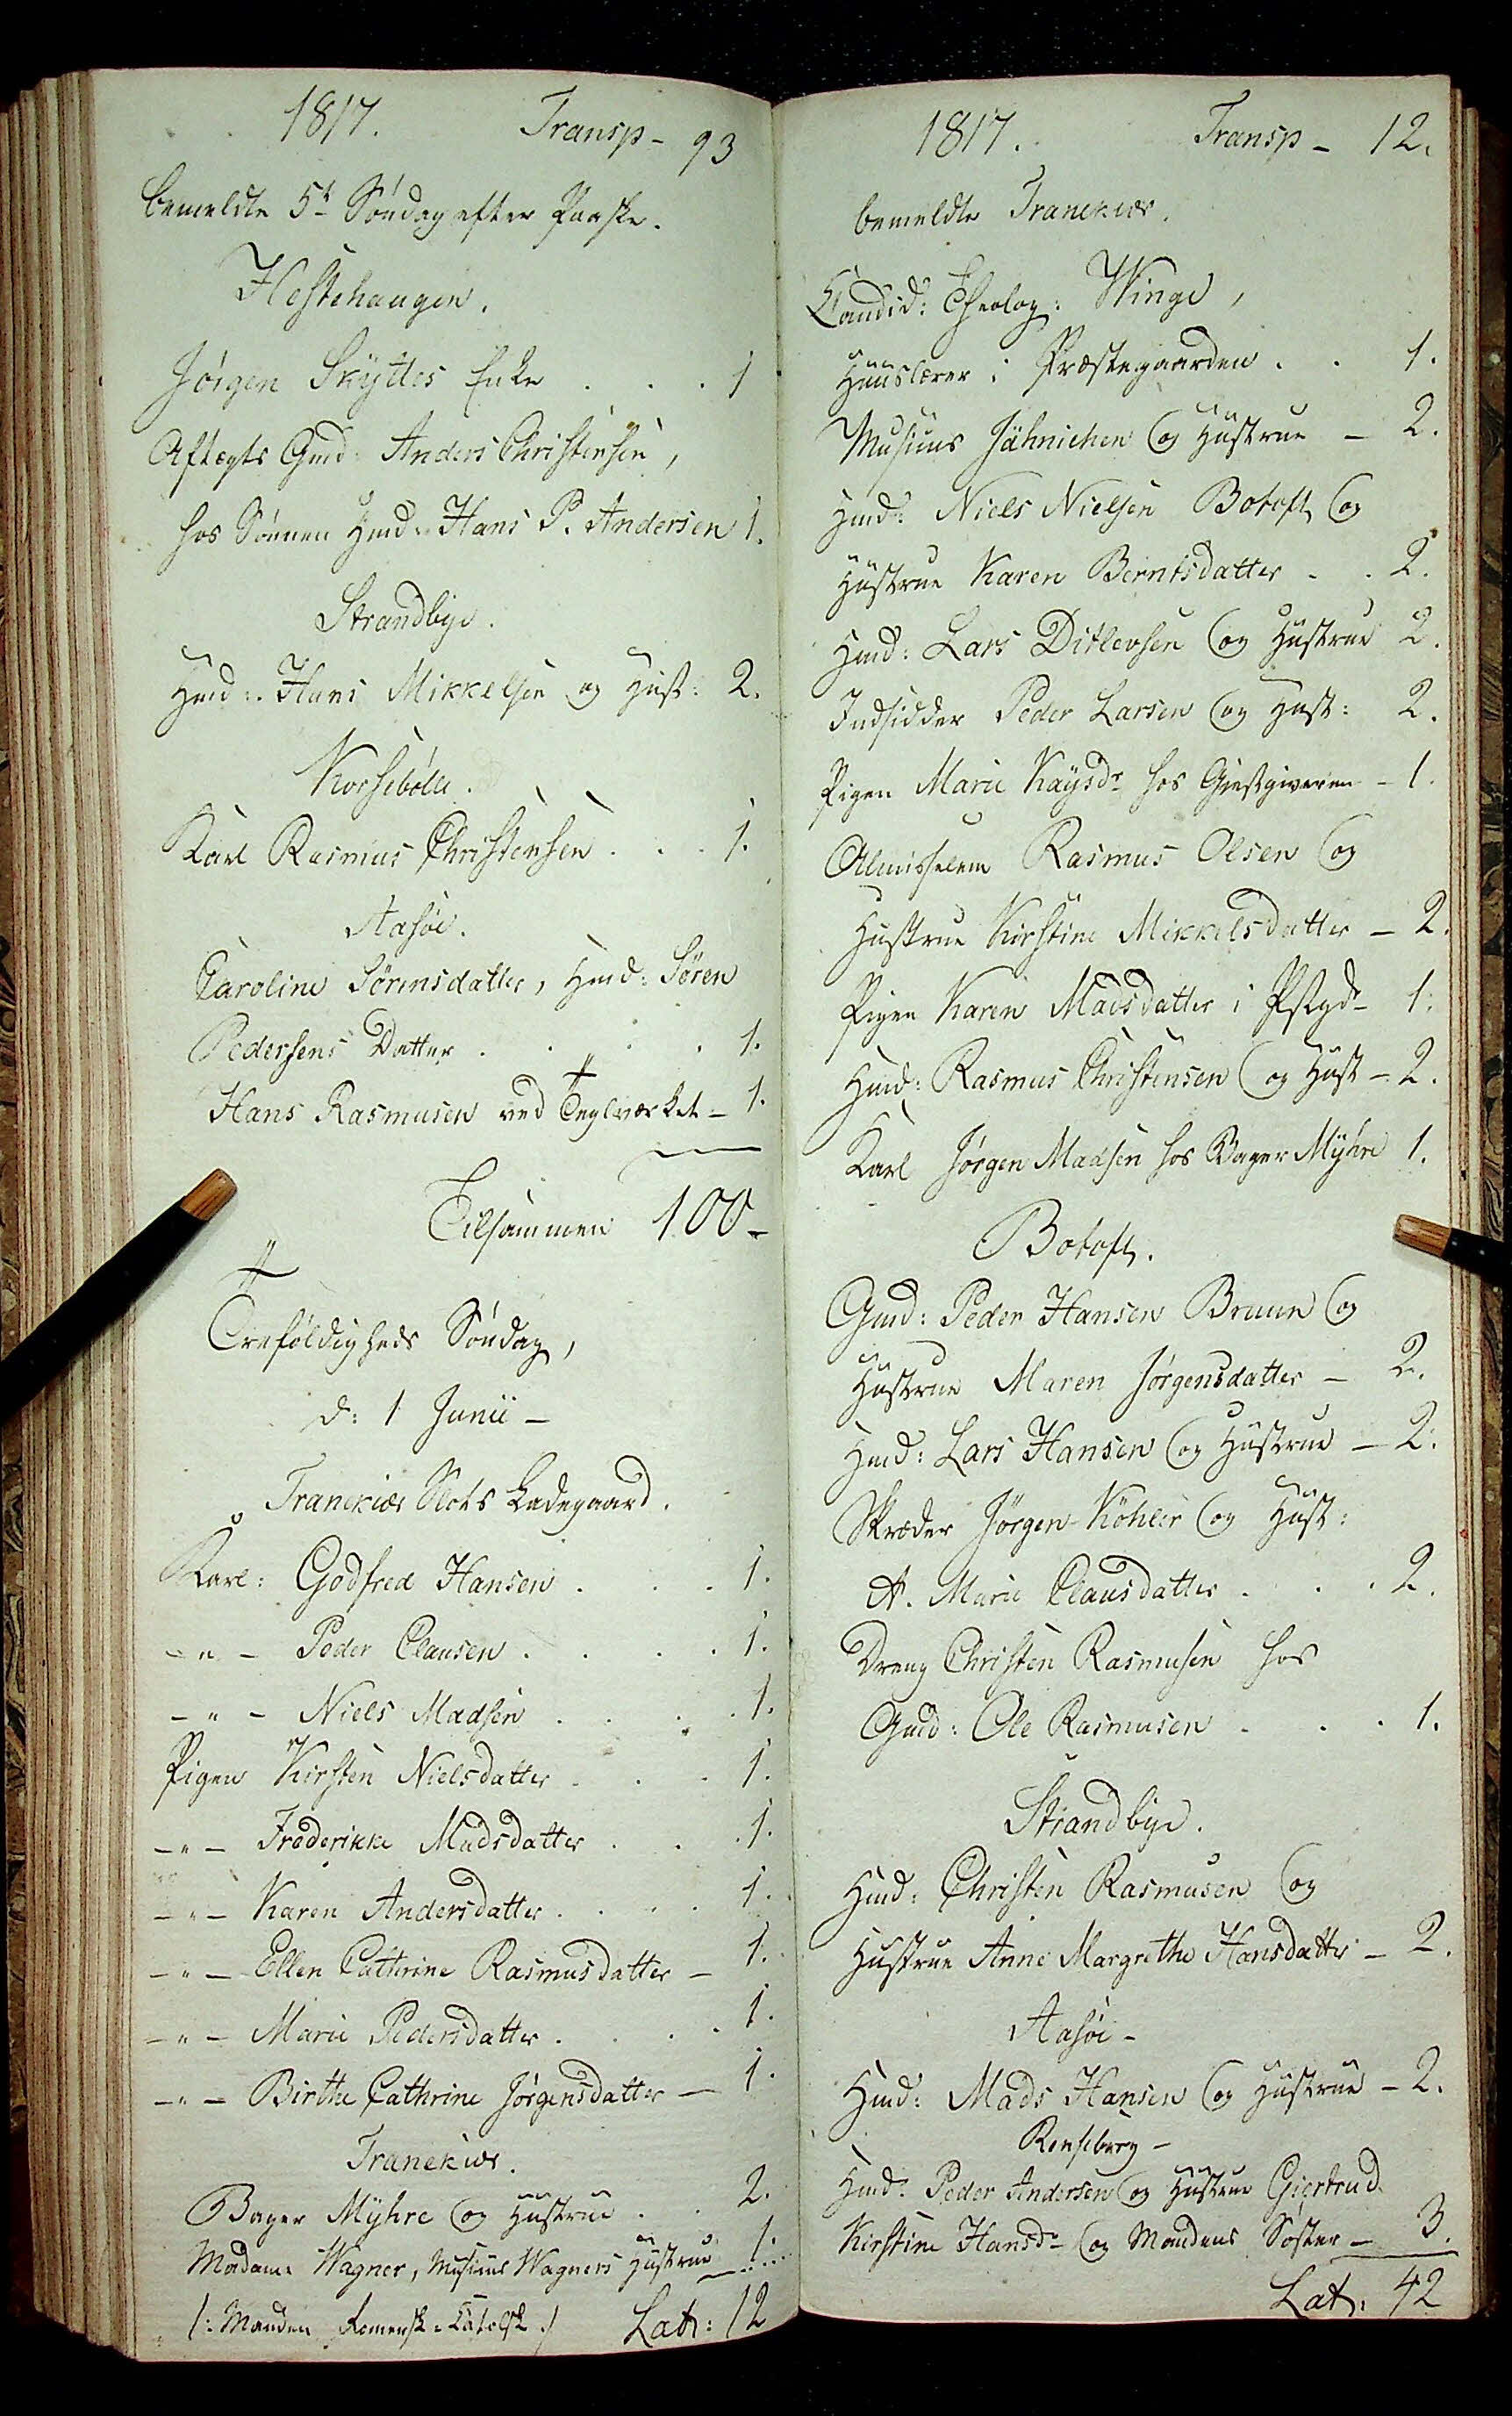1817.
Transp - 93
bemeldte 5te Søndag efter Paaske.
Hestehaugen.
Jørgen Skyttes Enke . . . 1
Aftægts Gmd. Anders Christensen
hos Sønnen Hmd: Hans P. Andersen 1.
Strandnye.
Hmd: Hans Mikkelsen og Hust: 2.
Korsebølle.
Karl Rasmus Christensen . . .1.
Aasøe.
Caroline Sørnsdatter, Hmd: Søren
Pedersens Datter . . . .1.
Hans Rasmusen ved Teglværket - 1.
Tilsammen 100-
Trefoldigheds Søndag
d: 1 Junii-
Tranekiær Slots Ladegaard.
Karl Godfred Hansen . . . 1.
-"- Peder Clausen . . . . 1.
-"- Niels Madsen . . . . 1.
Pigen Kirsten Nielsdatter . . . 1.
-"- Friderikke Madsdatter . . .1.
-"- Karen Andersdatter . . . . 1.
-"- Ellen Cathrine Rasmusdatter - 1.
-"- Marie Pedersdatter . . . . 1.
-"- Birthe Cathrine Jørgensdatter - 1.
Tranekiær
Bager Myhre og Hustrue . . 2.
Madame Wagner, Musicus Wagers Hustrue 1.
/: Manden Romersk Catolsk ./ Lat: 12
1817.
Transp - 12.
bemeldte Tranekiær
Candid: Theolog: Winge
Huuslærer i Præstegaarden . . 1.
Musicus Jahnichen og Hustrue - 2.
Hmd: Niels Nielsen Botoft og
Hustrue Karen Berntsdatter . . 2.
Hmd: Lars Ditlevsen og Hustrue 2.
Indsidder Peder Larsen og Hust: 2.
Pigen Marie Kaysdr hos Giestgiveren - 1.
Almisselem Rasmus Olsen og
Hustrue Kirstine Mikkelsdatter - 2
Pigen Karen Madsdatter i Pstgdr 1:
Hmd: Rasmus Christensen og Hust - 2.
Karl Jørgen Madsen hos Bager Muhre 1.
Botoft.
Gmd: Peder Hansens Bruun og
Hustrue Maren Jørgensdatter - 2.
Hmd: Lars Hansen og Hustrue - 2.
Skrædder Jørgen Køhler og Hust:
A. Marie Clausdatter . . . 2.
Dreng Christen Rasmusen hos
Gmd: Ole Rasmusen . . . 1.
Strandbye.
Hmd: Christen Rasmusen og
Hustrue Anne Margrethe Hansdatter - 2
Aasøe.
Hmd: Mads Hansens og Hustrue - 2.
Renseberg
Hmd: Peder Andersen og Hustrue Giertrud.
Kirstine Hansdr og Mandens Søster - 3
Lat: 42
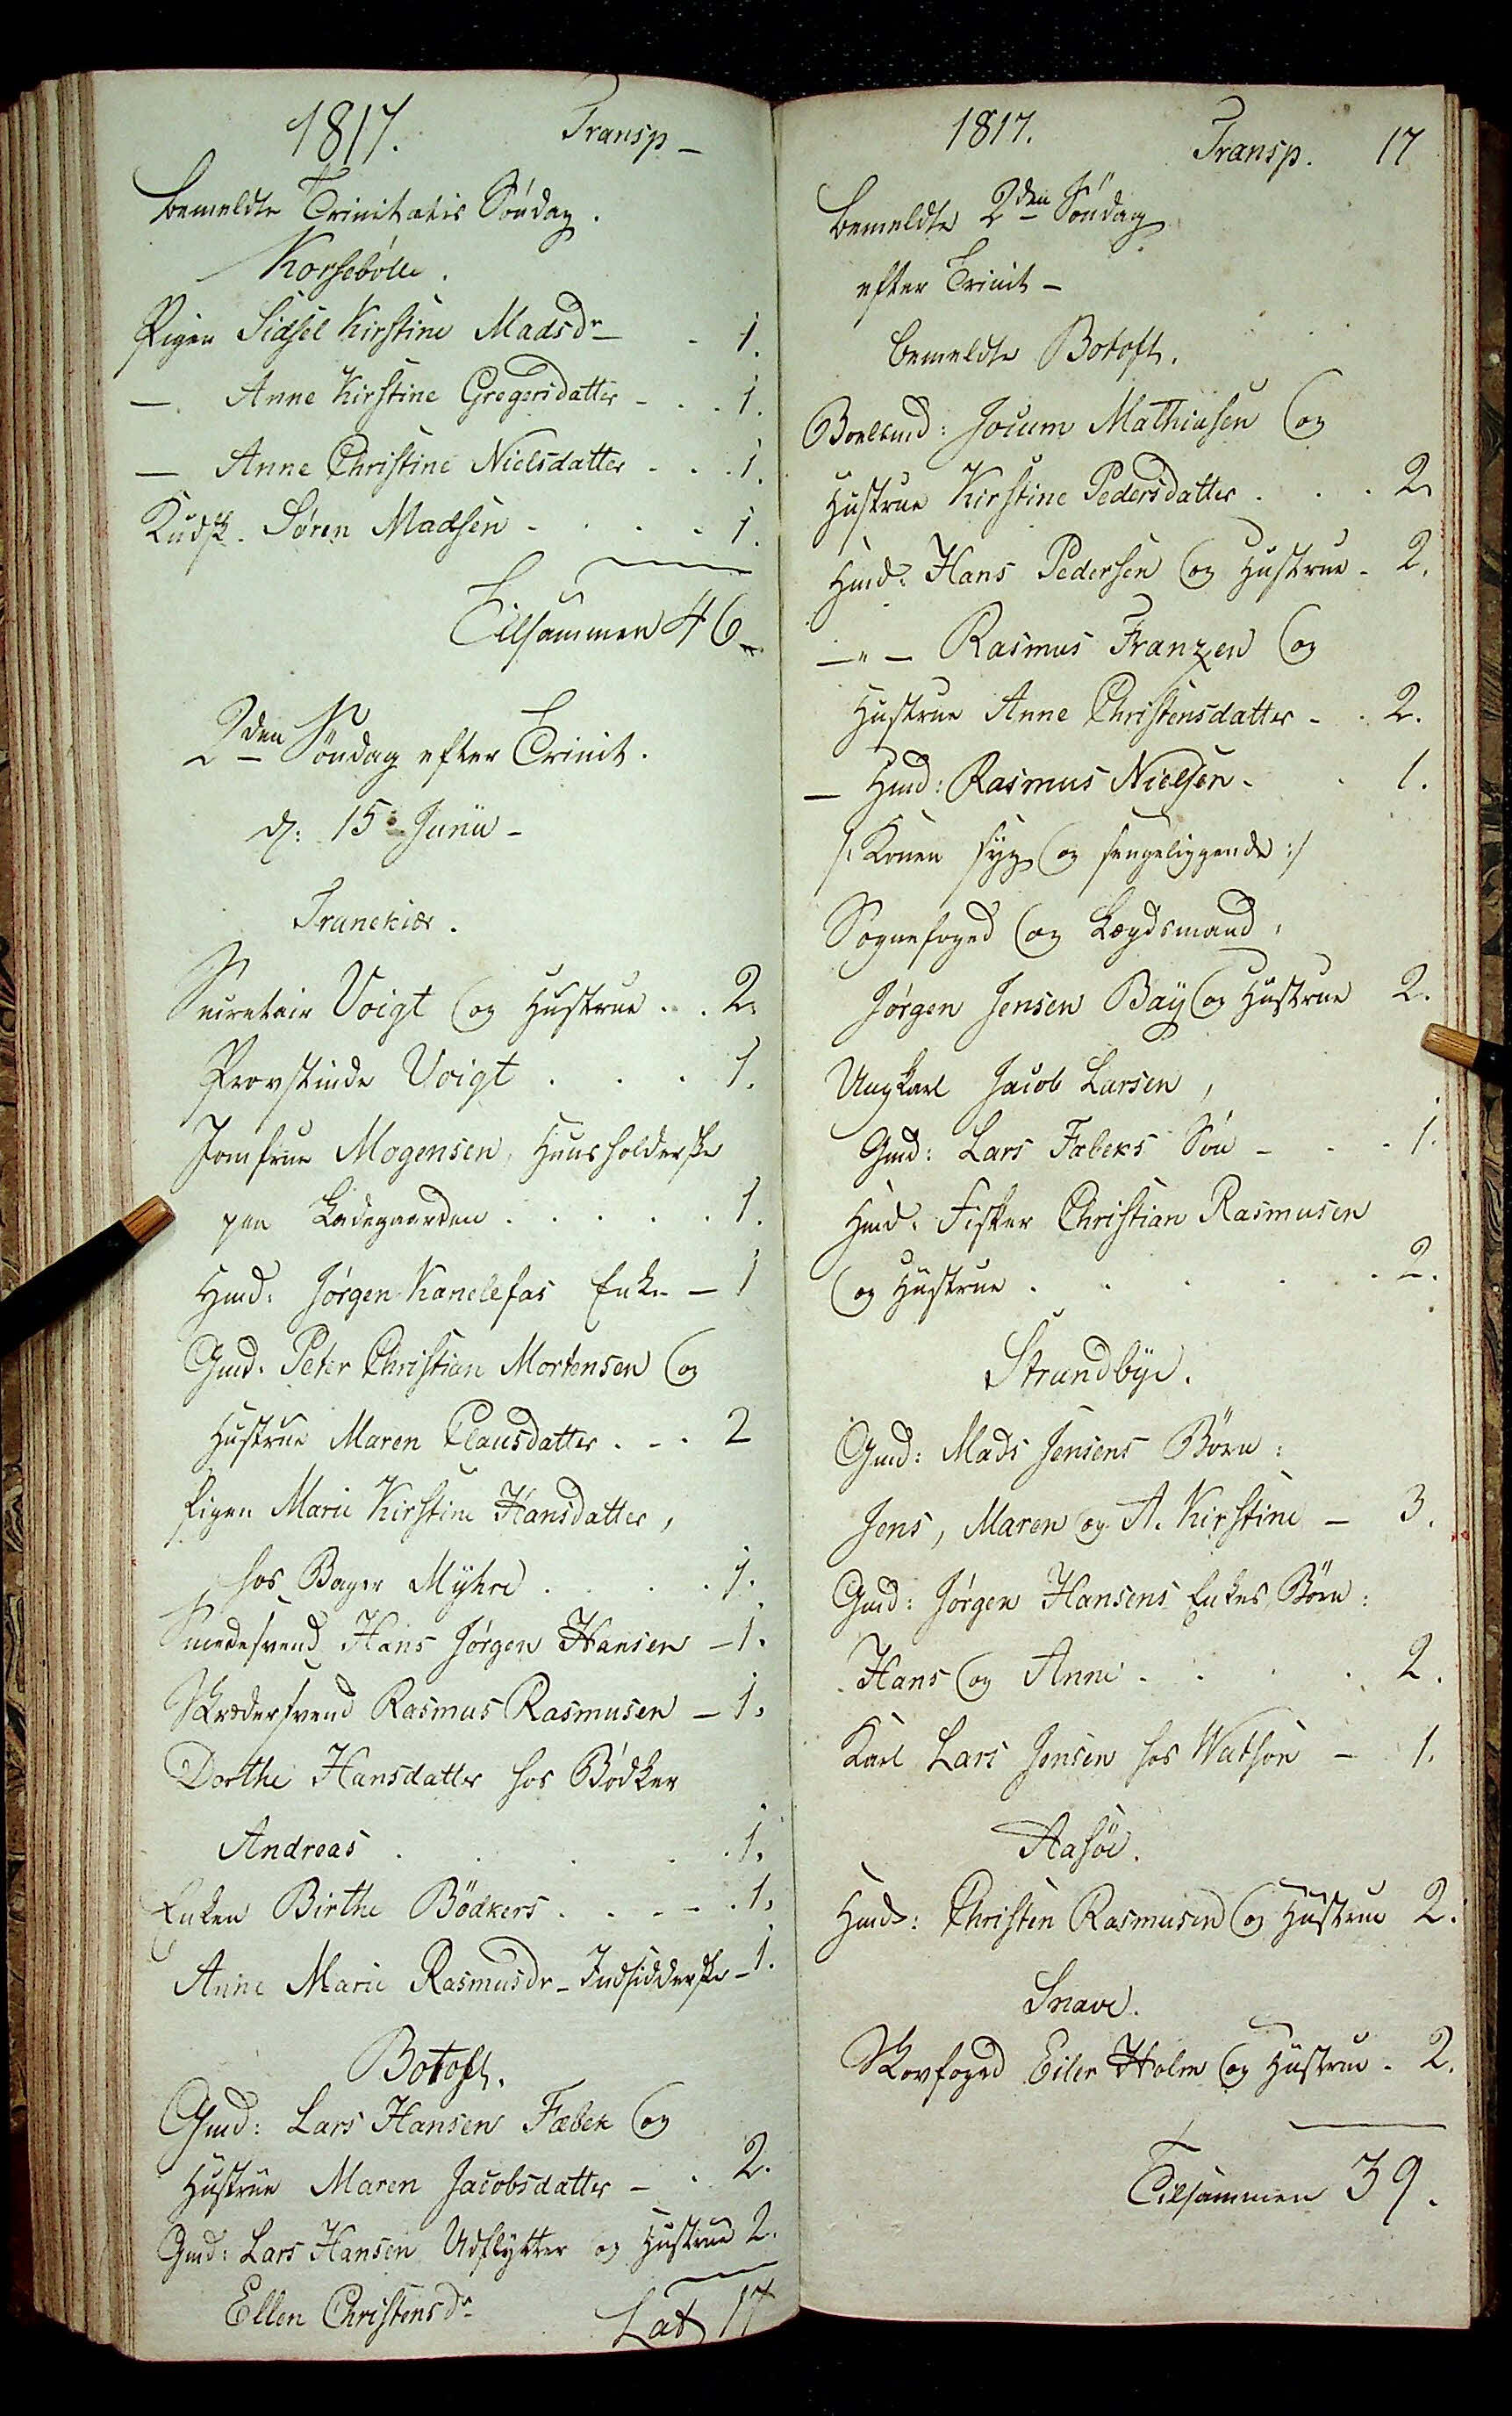1817.
Transp -
bemmeldte Trinitatis Søndag
Korsebølle.
Pigen Sidsel Kirstine Madsdr . 1.
- Anne Kirstine Gregersdatter - . . 1.
- Anne Christine Nielsdatter . . .  1.
Kudsk Søren Madsen . . . . 1.
Tilsammen 46-
2den Søndag efter Trinit.
d: 15 Junii-
Tranekiær.
Secretair Voigt og Hustrue . . 2.
Provstinde Voigt . . . 1.
Jomfrue Mogensen, Huusholderske
paa Ladegaarden . . . . . 1.
Hmd: Jørgen Kanelefas Enke - 1
Gmd: Peter Christian Mortensen og
Hustrue Maren Clausdatter . . . 2
Pigen Marie Kirstine Hansdatter,
hos Bager Myhre . . . . 1.
Smedesvend, Hans Jørgen Hansen - 1.
Skrædersvend Rasmus Rasmusen - 1.
Dorthe Hansdatter hos Bødker
Andreas . . . . . 1.
Enken Birthe Bødkers . . . . . 1.
Anne Marie Rasmusdr - Indsidderske - 1.
Botoft.
Gmd: Lars Hansen Fæbek og
Hustrue Maren Jacobsdatter - . 2.
Gmd: Lars Hansen Udflytter og Hustru 2.
Ellen Christensdr
Lat 17
1817.
Transp. 17
bemmeldte 2den Søndag
efter Trinit-
bemeldte Botoft.
Boelsmd: Jocum Mathiasen og
Hustrue Kirstine Pedersdatter . . . 2.
Hmd: Hans Pedersen og Hustrue . 2.
-"- Rasmus Franzen og
Hustrue Anne Christensdatter . . 2.
Hmd: Rasmus Nielsen . .  1.
/: Konen syg og sengeligende :/
Sognefoged og Lægdsmand
Jørgen Jensen Bay og Hustrue 2.
Ungkarl Jacob Larsen
Gmd: Lars Fæbeks Søn - . . 1.
Hmd: Fisker Christian Rasmusen
og Hustrue . . . . . . 2.
Strandbye.
Gmd: Mads Jensens Børn:
Jens, Maren og A. Kirstine - 3.
Gmd: Jørgen Hansens Enkes Børn:
Hans og Anne . . . . 2.
Karl Lars Jensen hos Watson - 1.
Aasøe.
Hmd: Christen Rasmusen og Hustrue 2.
Snave.
Skovfoged Eiler Holm og Hustrue . 2.
Tilsammen 39.